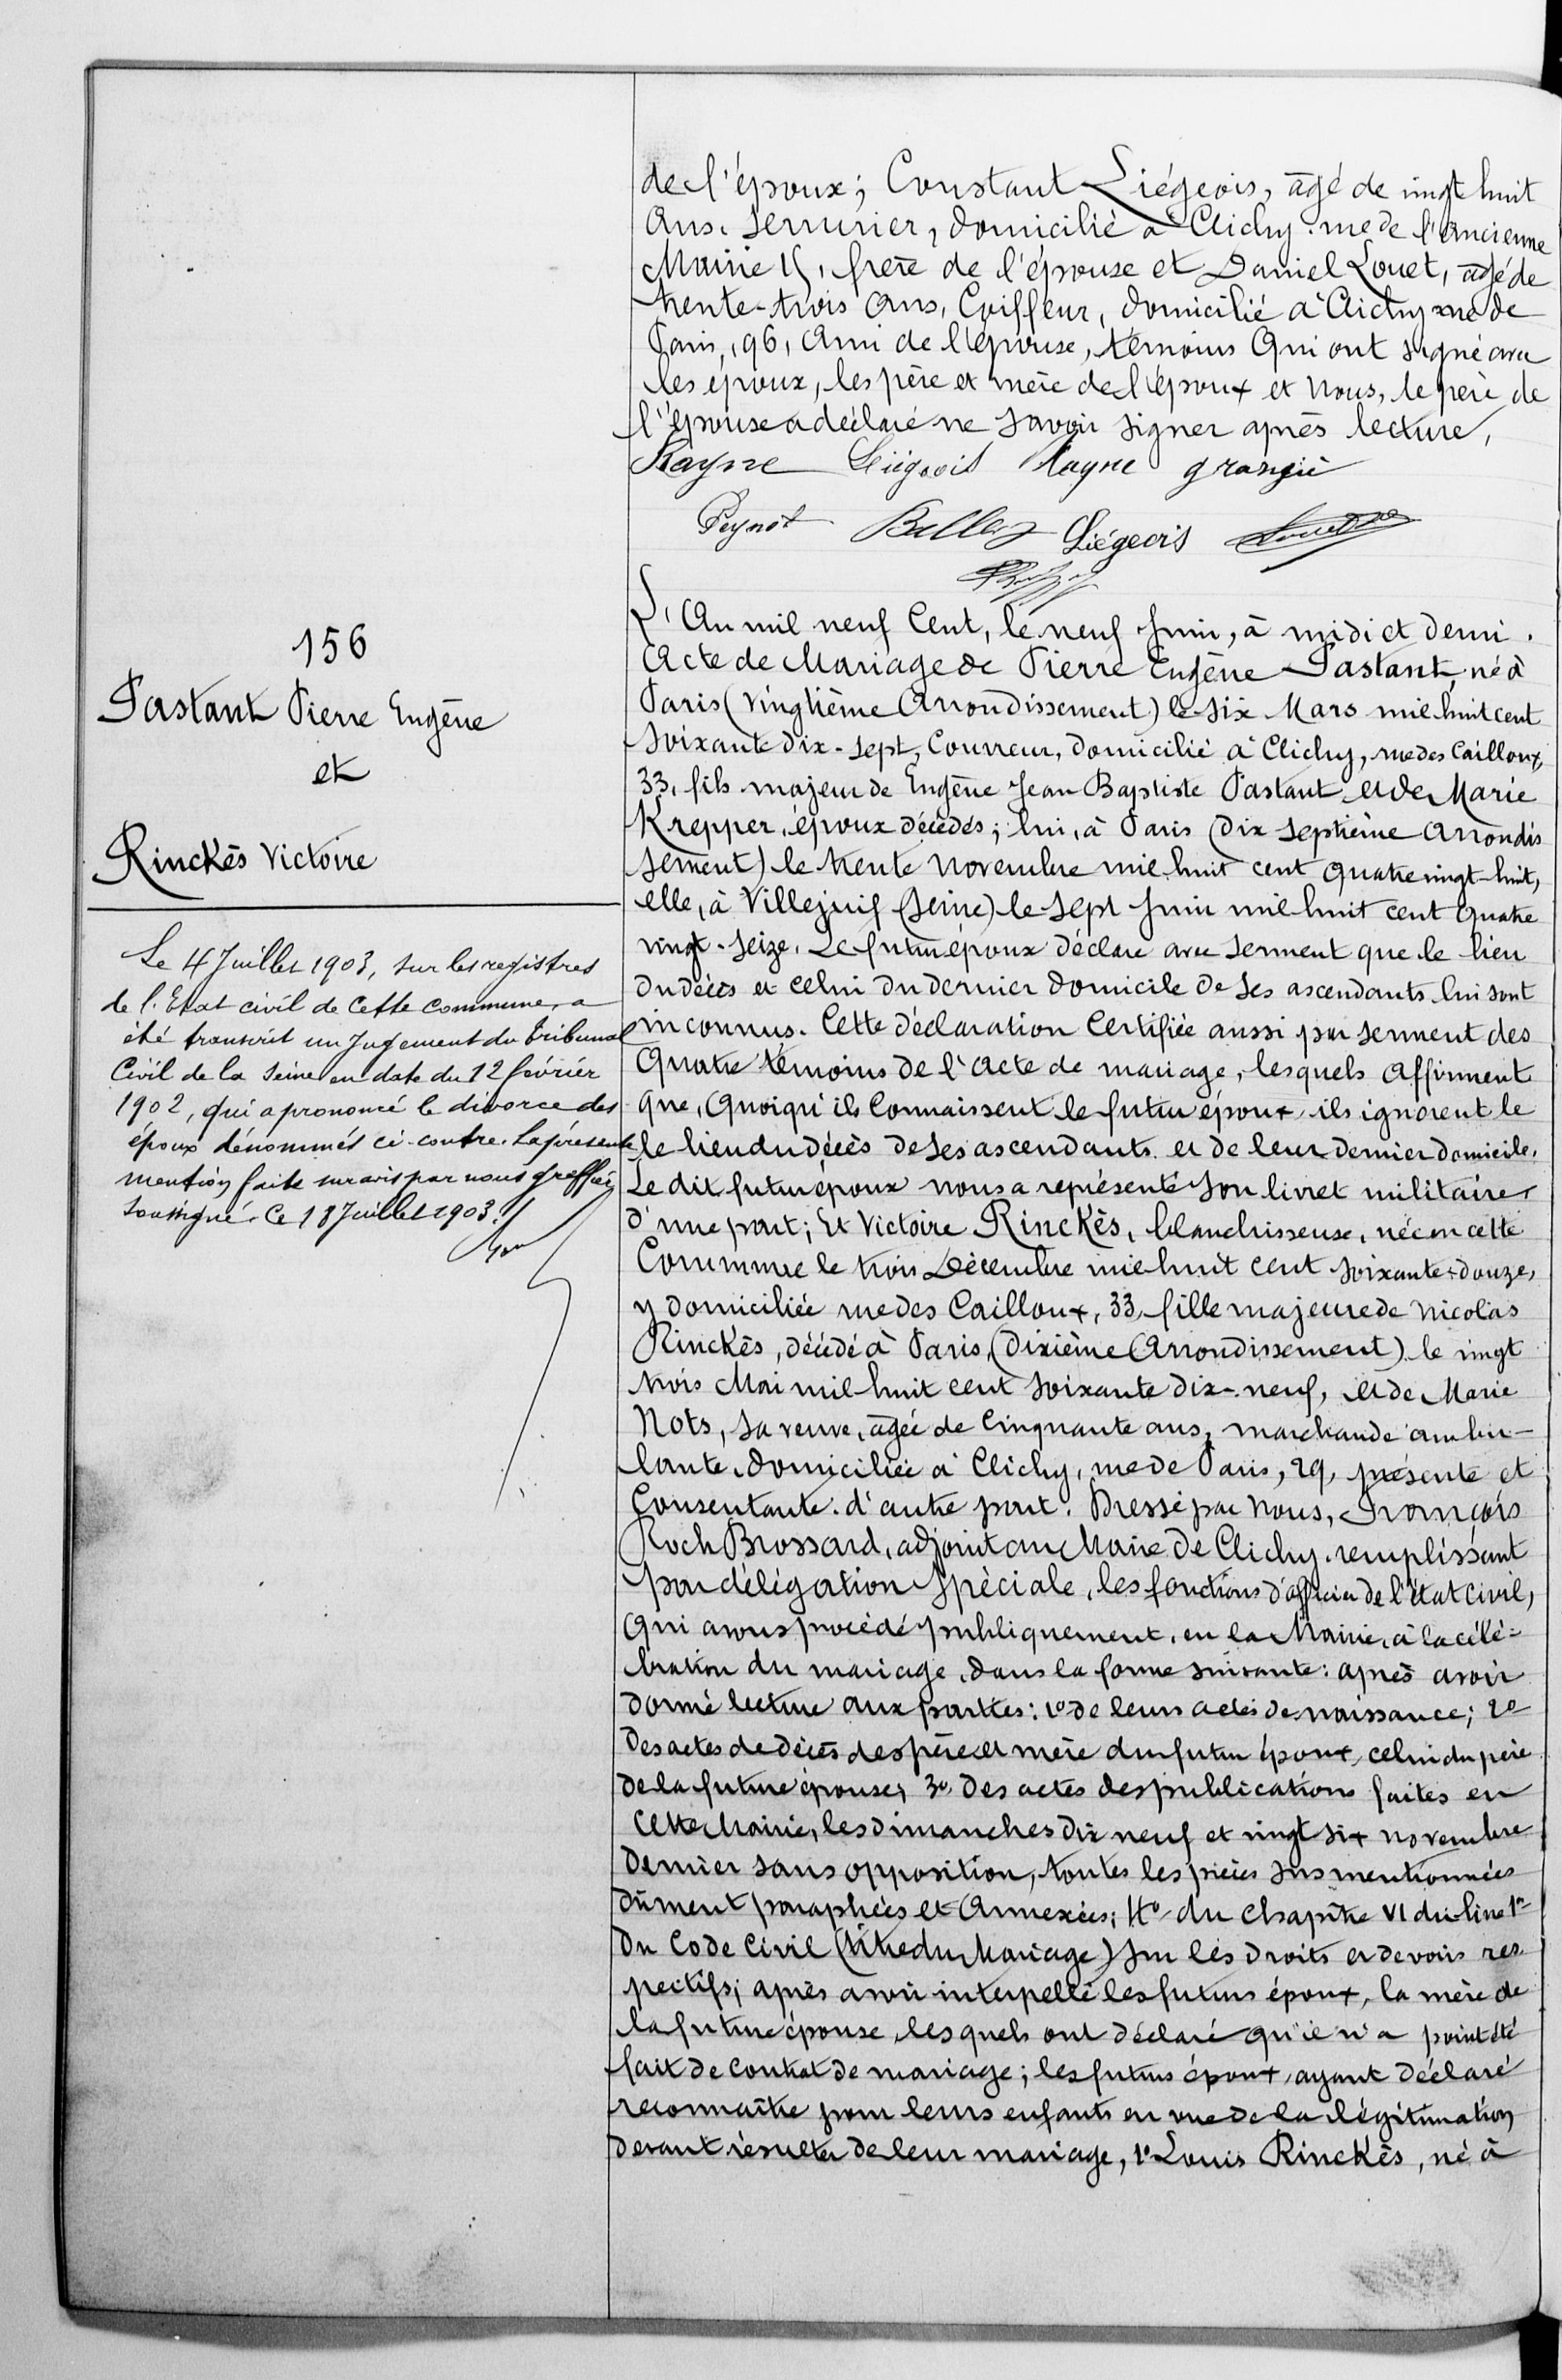de l'époux; Constant Liégois, âgé de vingt huit
ans, serrurier, domicilié à Clichy, rue de l'ancienne
Mairie 15, frère de l'épouse et Daniel Louet, âgé de
trente-trois ans, coiffeur, domicilié à Clichy rue de
Paris, 196, ami de l'épouse, témoins qui ont signé avec
les époux, les père et mère de l'époux et nous, le père de
l'épouse a déclaré ne savoir signer après lecture
156
Pastant Pierre Eugène
et
Rinckès Victoire
L'an mil neuf cent, le neuf juin, à midi et demi.
Acte de mariage de Pierre Eugène Pastant, né à
Paris (vingtième arrondissement) le six mars mil huit cent
soixante dix-sept, courreur, domicilié à Clichy, rue des Cailloux
33, fils majeur de Eugène Jean Baptiste Pastant et de Marie
Krepper, époux décédés; lui, à Paris (dix septième arrondis
sement) le trente novembre mil huit cent quatre vingt-huit,
elle à Villejuif (Seine) le sept juin mil huit cent quatre
vingt-seize, le futur époux déclare avec serment que le lieu
du décès et celui du dernier domicile de ses ascendants lui sont
inconnus. Cette déclaration certifiée aussi sur serment des
quatre témoins de l'acte de mariage, lesquels affirment
que, quoiqu'ils connaissent le futur époux ils ignorent le
le lieu du décès de ses ascendants et de leur dernier domicile
Le dit futur époux nous a représenté son livret militaire
d'une part; Et Victoire Rinckès, blanchisseuse, née en cette
commune le trois décembre mil huit cent soixante douze,
y domiciliée rue des Cailloux, 33, fille majeure de nicolas
Rinckès, décédé à Paris (dixième arrondissement) le vingt
trois mai mil huit cent soixante dix-neuf, et de Marie
Nots, sa veuve, âgée de cinquante ans, marchande ambu-
lante, domiciliée à Clichy, rue de PAris, 29, présente et
consentante, d'autre part. Dressé par nous, François
Roch Brossard, adjoint au Maire de Clichy, remplissant
par délégation spéciale, les fonctions d'officier de l'état civil,
qui avons procédé publiquement, en la Mairie,) la célé-
bration du mariage, dans la forme suivante: après avoir
donné lecture aux parties: 1° de leurs actes de naissance; 2°
les actes de décès des père et mère du futur époux, celui du père
de la future épouse, 3° des actes des publications faites en
cette Mairie, les dimanches dix neuf et vingt six novembre
dernier sans opposition, toutes les pièces susmentionnées
dûment paraphées et annexées: 4° du chapitre VI du livre 1er
du code civil (titre du mariage) sur les droits et devoirs res
pectifs après avoir interpellé les futurs époux, la mère de
la future épouse lesquels ont déclaré qu'il n'a point été
fait de contrat de mariage; les futurs époux, ayant déclaré
reconnaître pour leurs enfants en vue de la légitimtion
devant résulter de leur mariage. 1° Louis Rickès, né à
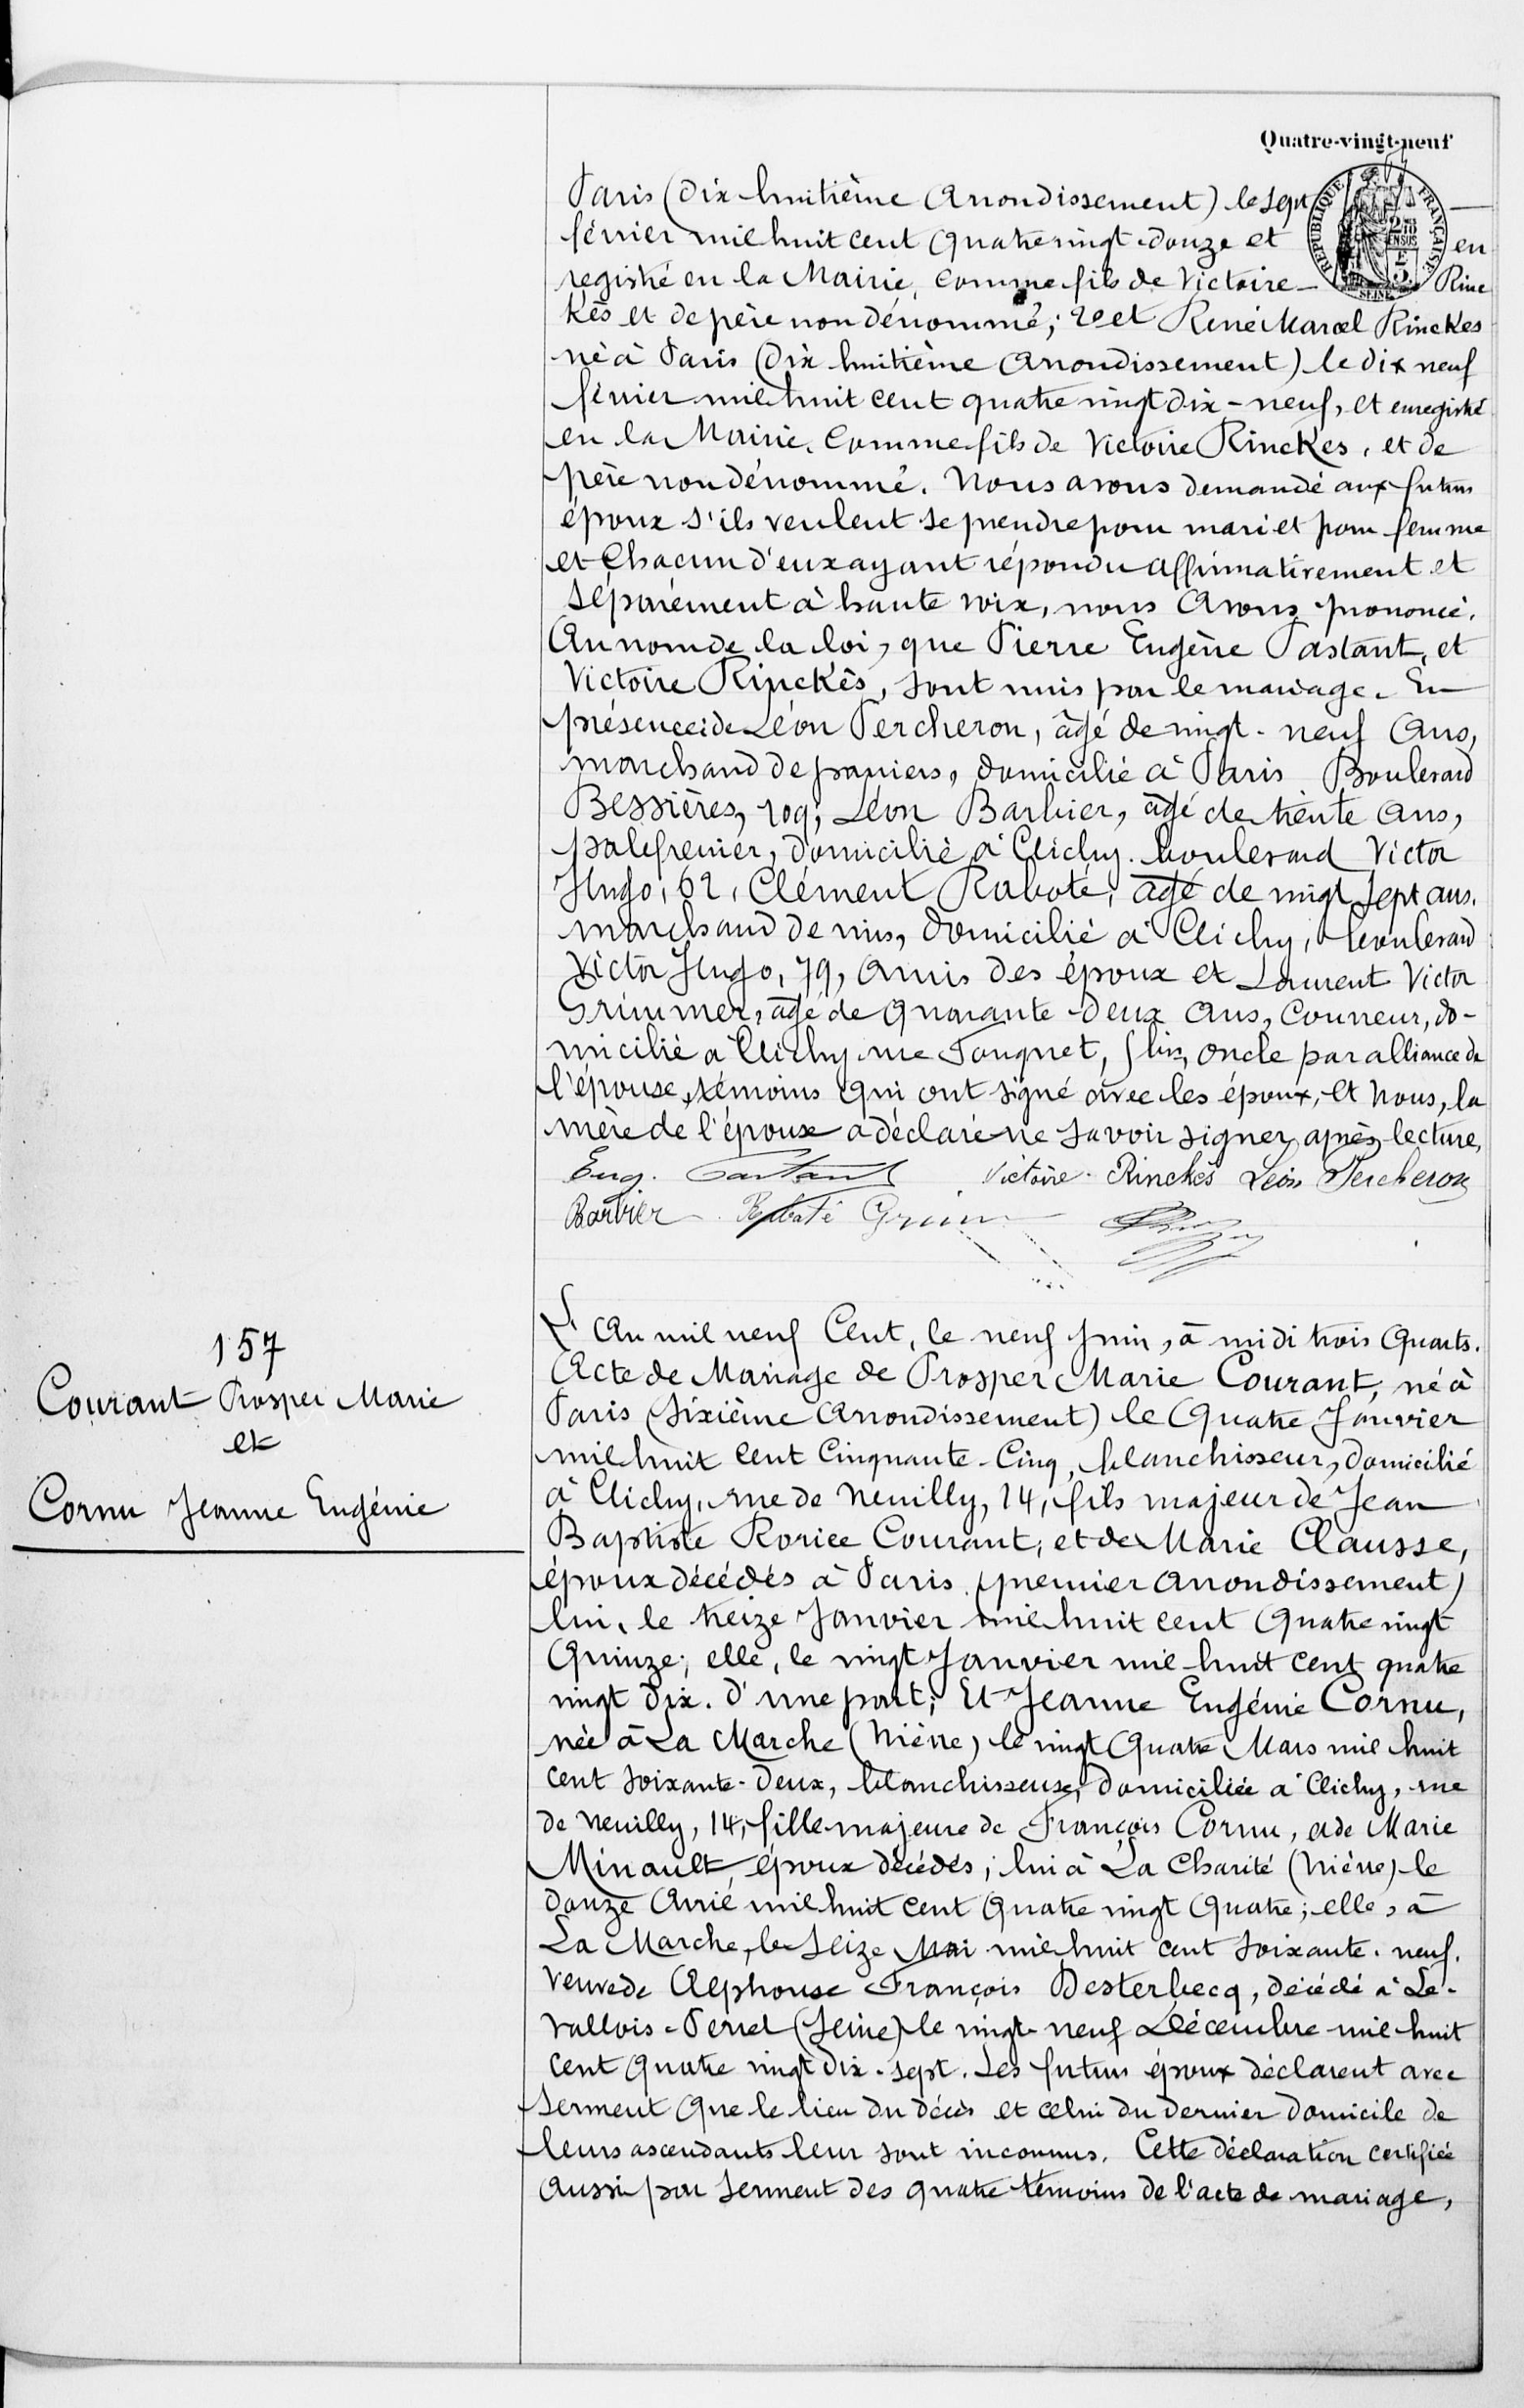Paris (dix huitième arrondissement) le sept
février mil huit cent quatre vingt douze et en
registre en la Mairie, comme fil de Victoire Rinck
kès et de père non dénommé; 2° et René Marcel Rinckers
né à Paris (dix huitième arrondissement) le dix neuf
février mil huit cent quatre vingt dix-neuf, et enregistré
en la Mairie, comme fils de Victoire Rinckes, et de
père non dénommé. Nous avons demandé aux futurs
époux s'ils veulent se prendre pour mari et pour femme
et chacun d'eux ayant répondu affirmativement et
séparément à haute voix, nous avons prononcé,
au nom de la loi, que Pierre Eugène Pastant et
Victoire Rinckès, sont unis par le mariage. En
présence de Léon Percheron, âgé de vingt-neuf ans,
marchand de paniers, domicilié à Paris Boulevard
Bessières, 109, Léon Barbier, âgé de trente ans,
palfrenier, domicilié à Clichy, boulevard Victor
Hugo, 62, Clément Raboté, agé de vingt sept ans,
marchand de vins, domicilié à Clichy, boulevard
Victor Hugo, 79, amis des époux et Laurent Victor
Grimmer, agé de quarante-deux ans, courreur, do-
micilié à Clichy rue Faupret, 5 bis, oncle par alliance de
l'épouse, témoins qui ont signé avec les époux, et nous, la
mère de l'époux a déclaré ne savoir signer après lecture
157
Courant Prosper Marie
et
Cornu Jeanne Eugénie
L'an mil neuf cent, le neuf juin, à midi trois quarts
acte de mariage de Prosper Marie Courant né à
Paris (Sixième arrondissement) le quatre Janvier
mil huit cent cinquante-cinq, blanchisseur, domicilié
à Clichy, rue de Neuilly, 14, fils majeur de Jean
Baptiste Rorice Courant,, et de Marie Clausse,
époux décédés à Paris (premier arrondissement)
lui, le treize janvier mil huit cent quatre vingt
quinze; elle le vingt janvier mil huit cent quatre
vingt dix. D'une part; Et Jeanne Eugénie Cornu,
née à La Marche (Nièvre) le vingt quatre Mars mil huit
cent soixante-deux, blanchisseuse, domiciliée à Clichy, rue
de Neuilly, 14, fille majeure de François Cornu, et de Marie
Minault, époux déccédés; lui à La Charité (Nièvre) le
douze avril mil huit cent quatre vingt quatre; elle, à
La Marche, le seize mai mil huit cent soixante neuf,
veuve de Alphonse François Desterbecq, décédé à Le-
Vallois-Perret (Seine) le vingt-neuf décembre mil huit
cent quatre vingt dix-sept. Les futurs époux déclarent avec
serment que le lieu du décès et celui du dernier domicile de
leurs ascendants leur sont inconnus. Cette déclaration certifiée
aussi par serment des quatre témoins de l'acte de mariage,